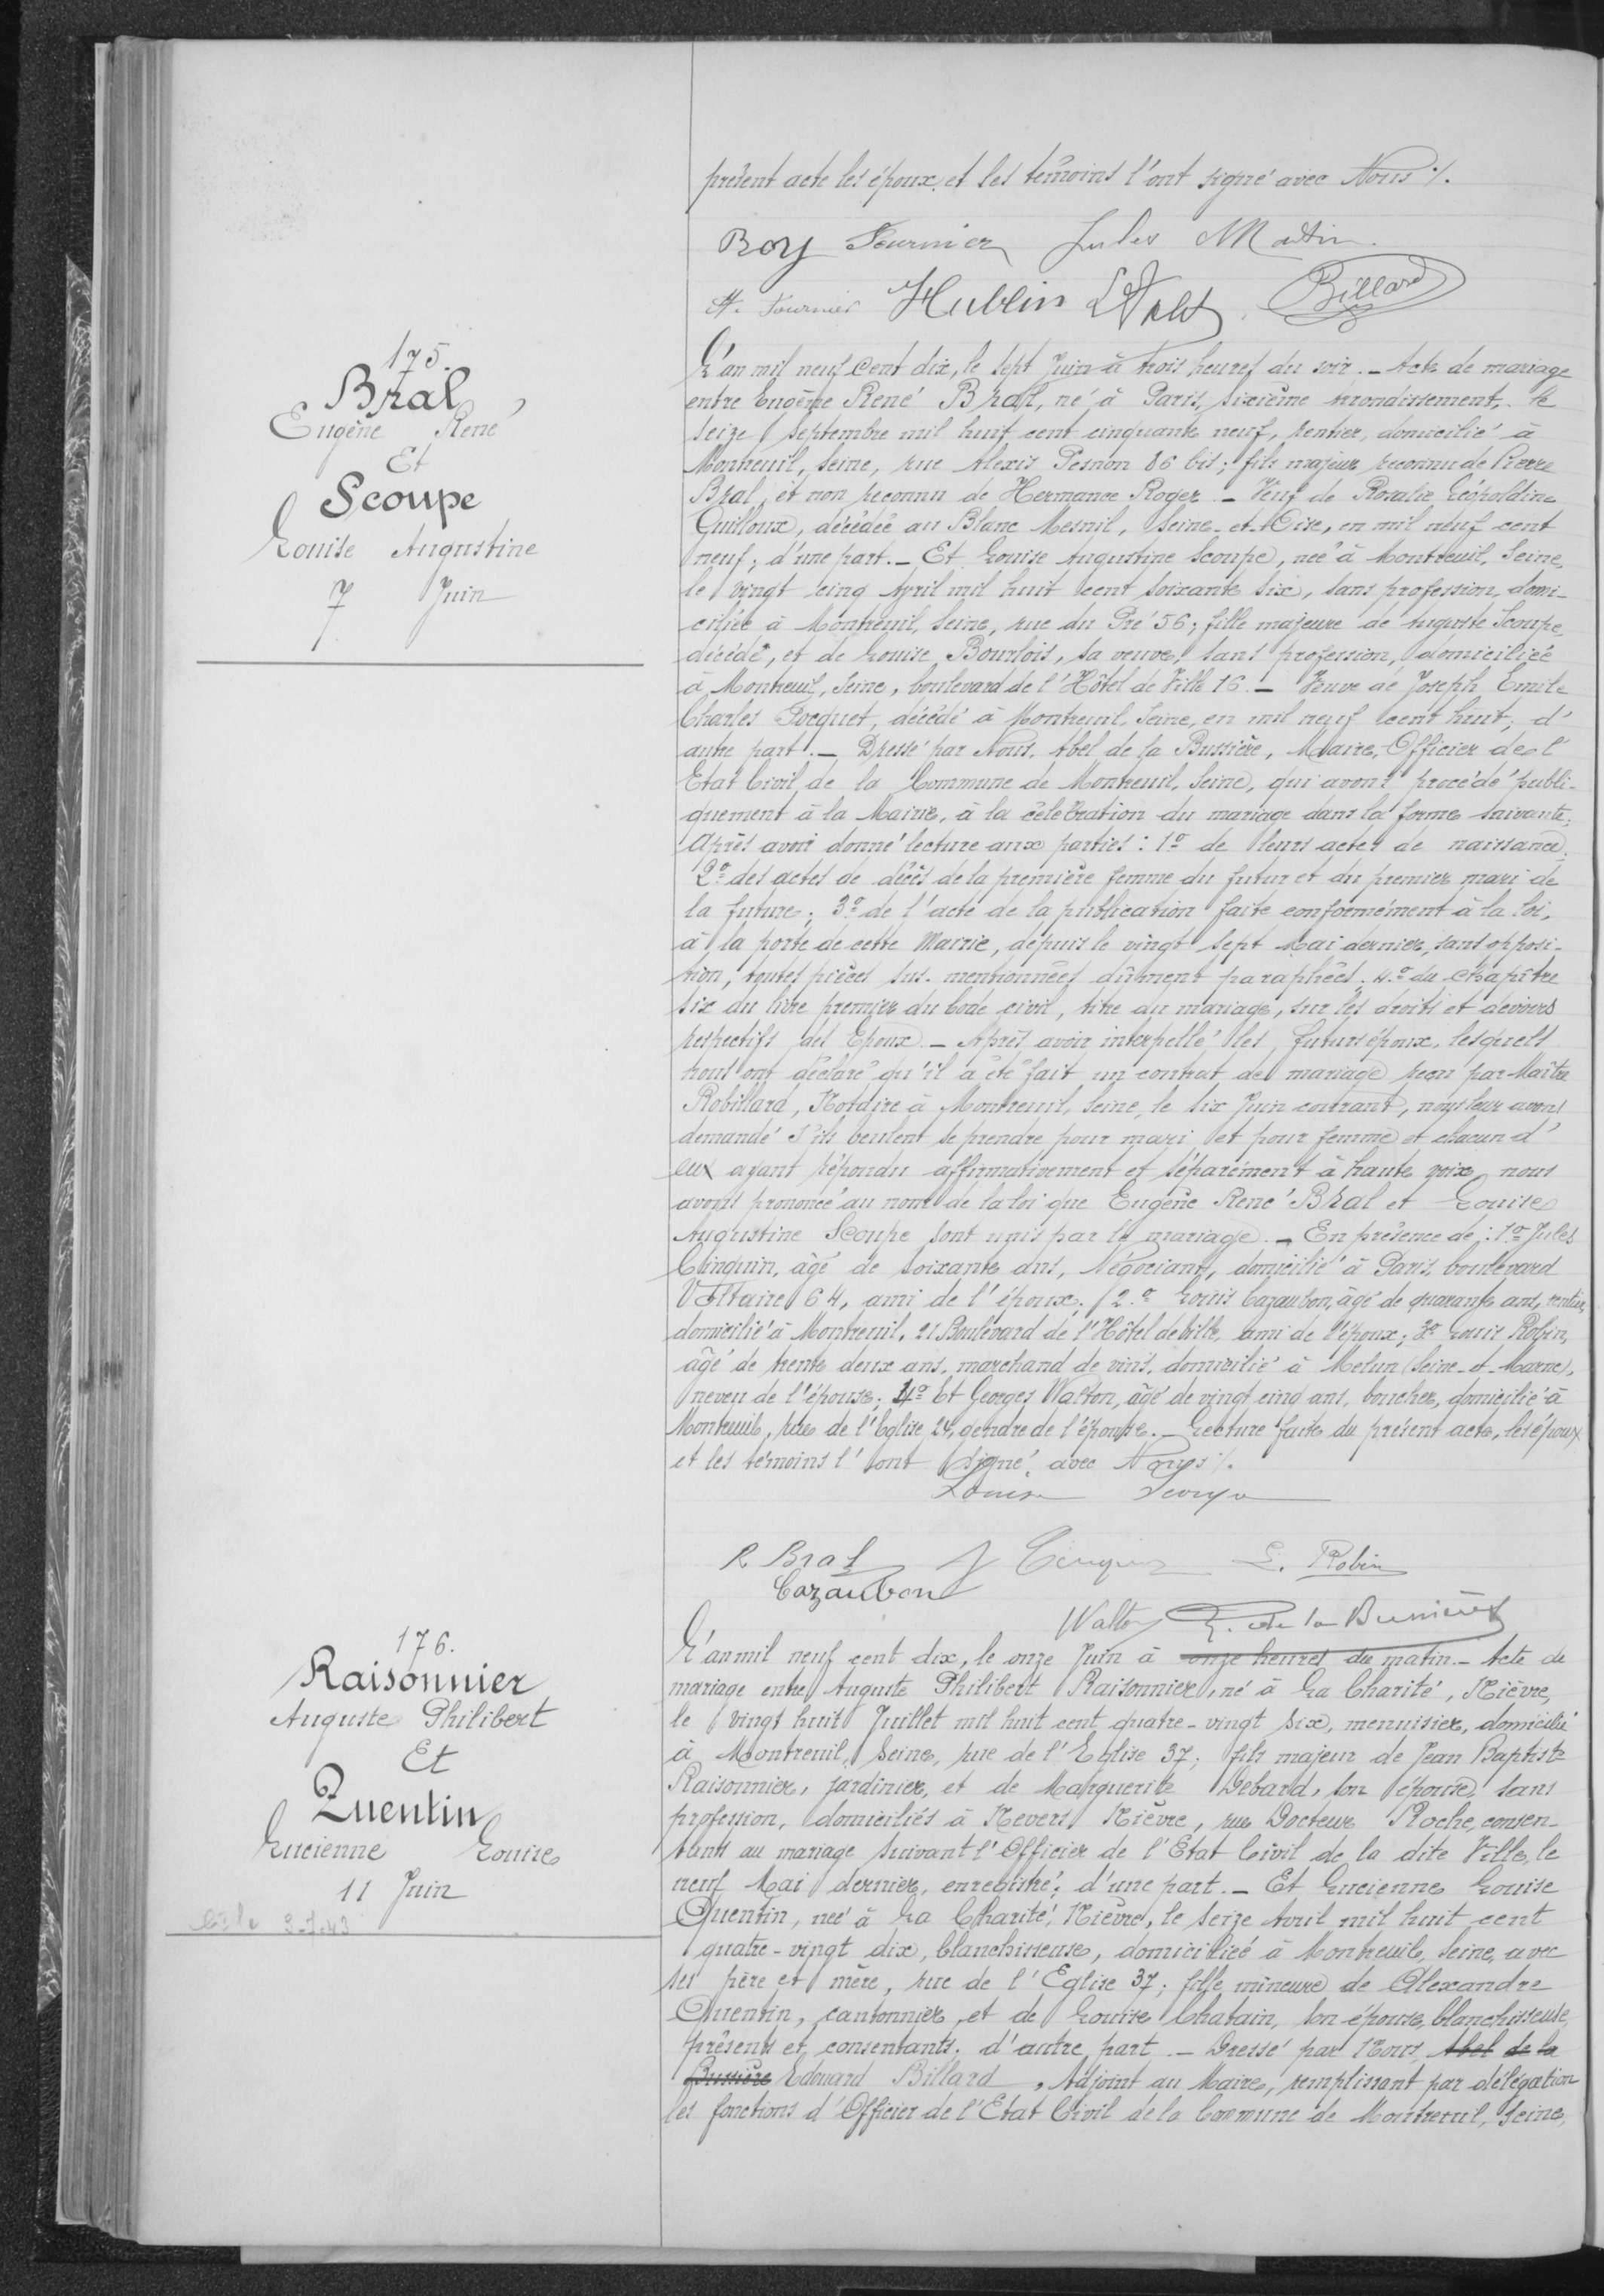175.
Bral
Eugène René
Et
Scoupe
Louise Augustine
7 Juin
L'an mil neuf cent dix, le sept Juin à trois heures du soir-Acte de mariage
entre Eugène René Bral, né à Paris, sixième arrondissement, le
seize septembre mil huit cent cinquante neuf, tennier, domicilié à
Montreuil, seine, rue Alexis Peson 86 bis; fils majeur reconnu de Pierre
Bral, et non reconnu de Hermance Roger-Veuf de Rosalie Léopoldine
Emilloux, décédée au Blanc Mesnil, Seine et oise, en mil neuf cent
neuf; d'une part.-Et Louise Augustine Scoupe, née à Montreuil, seine,
le vingt cinq Avril mil huit cent soixante six, sans profession, domi-
ciliée à Montreuil, Seine, rue du Pré 56; fille majeure de Auguste Scoupe,
décédé, et de Louise Bourlois, sa veuve, sans profession, domiciliée
à Montreuil, Seine, boulevard de l'Hîtel de Ville 16-Veuve de Joseph Emile
Charles Focquet, décédé à Montreuil, Seine, en mil neuf cent huit, d'
autre part .-Dressé par Nous, Abel de la Bussière, Maire, Officier de l'
Etat Civil de la Commune de Montreuil, Seine, qui avons procédé publi-
quement à la Mairie, à la célébration du mariage dans la forme suivante;
Après avoir donné lecture aux parties: 1°-de leurs actes de naissance,
2°-des actes de décès de la première femme du futur et des premier mari de
la future; 3°-de l'acte de la publication faite conformément à la loi,
à la porte de cette Mairie, depuis le vingt sept Mai dernier, sans opposi-
tion, toutes pièces sus-mentionnées dûment paraphées. 4°: du chapitre
six du livre premier du Code civil, titre du mariage, sur les droits et devoirs
respectifs des Epoux-Après avoir interpellé les futurs époux, lesquels
nous ont déclaré qu'il a été fait un contrat de mariage reçu par Maître
Robillard, notaire à Montreuil, Seine, le six Juin courant, nous leur avons
demandé s'ils veulent se prendre pour mari et pour femme et chacun d'
eux ayant répondu affirmativement et séparément à haute voix, nous
avons prononcé au nom de la loi que Eugène René Bral et Louise
Augustine Scoupe sont unis par le mariage-En présence de: 1°: Jules
Clinquin, âgé de soixante ans, Négociant, domicilié à Paris, boulevard
Voltaire 64, ami de l'époux; 2°/ Louis Cazaubon, âgé de quarante ans, rentier,
domicilié à Montreuil, 21 Boulevard de l'Hôtel de Ville, ami de l'époux; 3° Louis Robin,
âgé de trente deux ans, marchand de vins, domicilié à Melun (Seine-et-Marne),
neveu de l'époux; 4°: Et Georges Walton, âgé de vingt cinq ans, boucher, domicilié à
Montreuil, rue de l'Eglise 24, gendre l'épouse.-Lecture faite du présent acte, les époux
et les témoins l' ont signé avec Nous ./.
176
Raisonnier
Auguste Philibert
Et
Quentin
Lucienne Louise
11 Juin
L'an mil neuf cent dix, le onze Juin à onze heures du matin.-Acte de
mariage entre Auguste Philibert Raisonnier, né à la Charité, Nièvre,
le vingt huit Juillet, mil huit cent quatre-vingt dix, menuisier, domicilié
à Montreuil, Seine, rue de l'Eglise 37; fils majeur de Jean Baptiste
Raisonnier, jardinier, et de Marguerite Debard, son épouse, sans
profession, domiciliés à Nvers Nièvre, rue Docteur Roche, consen-
tants au mariage suivant l'Officier de l'Etat Civil de la dite Ville, le
neuf Mai dernier, enregistré d'une part .-Et Lucienne Louise
Quentin, née à la Charité, Nièvre, le seize Avril mil huit cent
quatre-vingt dix, blanchisseuse, domiciliée à Montreuil, Seine, avec
ses père et mère, rue de l'Eglise 37; fille mineure de Alexandre
Quentin, cantonnier, et de Louise Chatain, son épouse, blanchisseuse,
présents et consentants, d'autre part .-Dressé par Nous,
Edouard Billard, Adjoint au Maire, remplissant par délégation
les fonctions d'Officier de l'Etat Civil de la Commune de Montreuil, Seine,
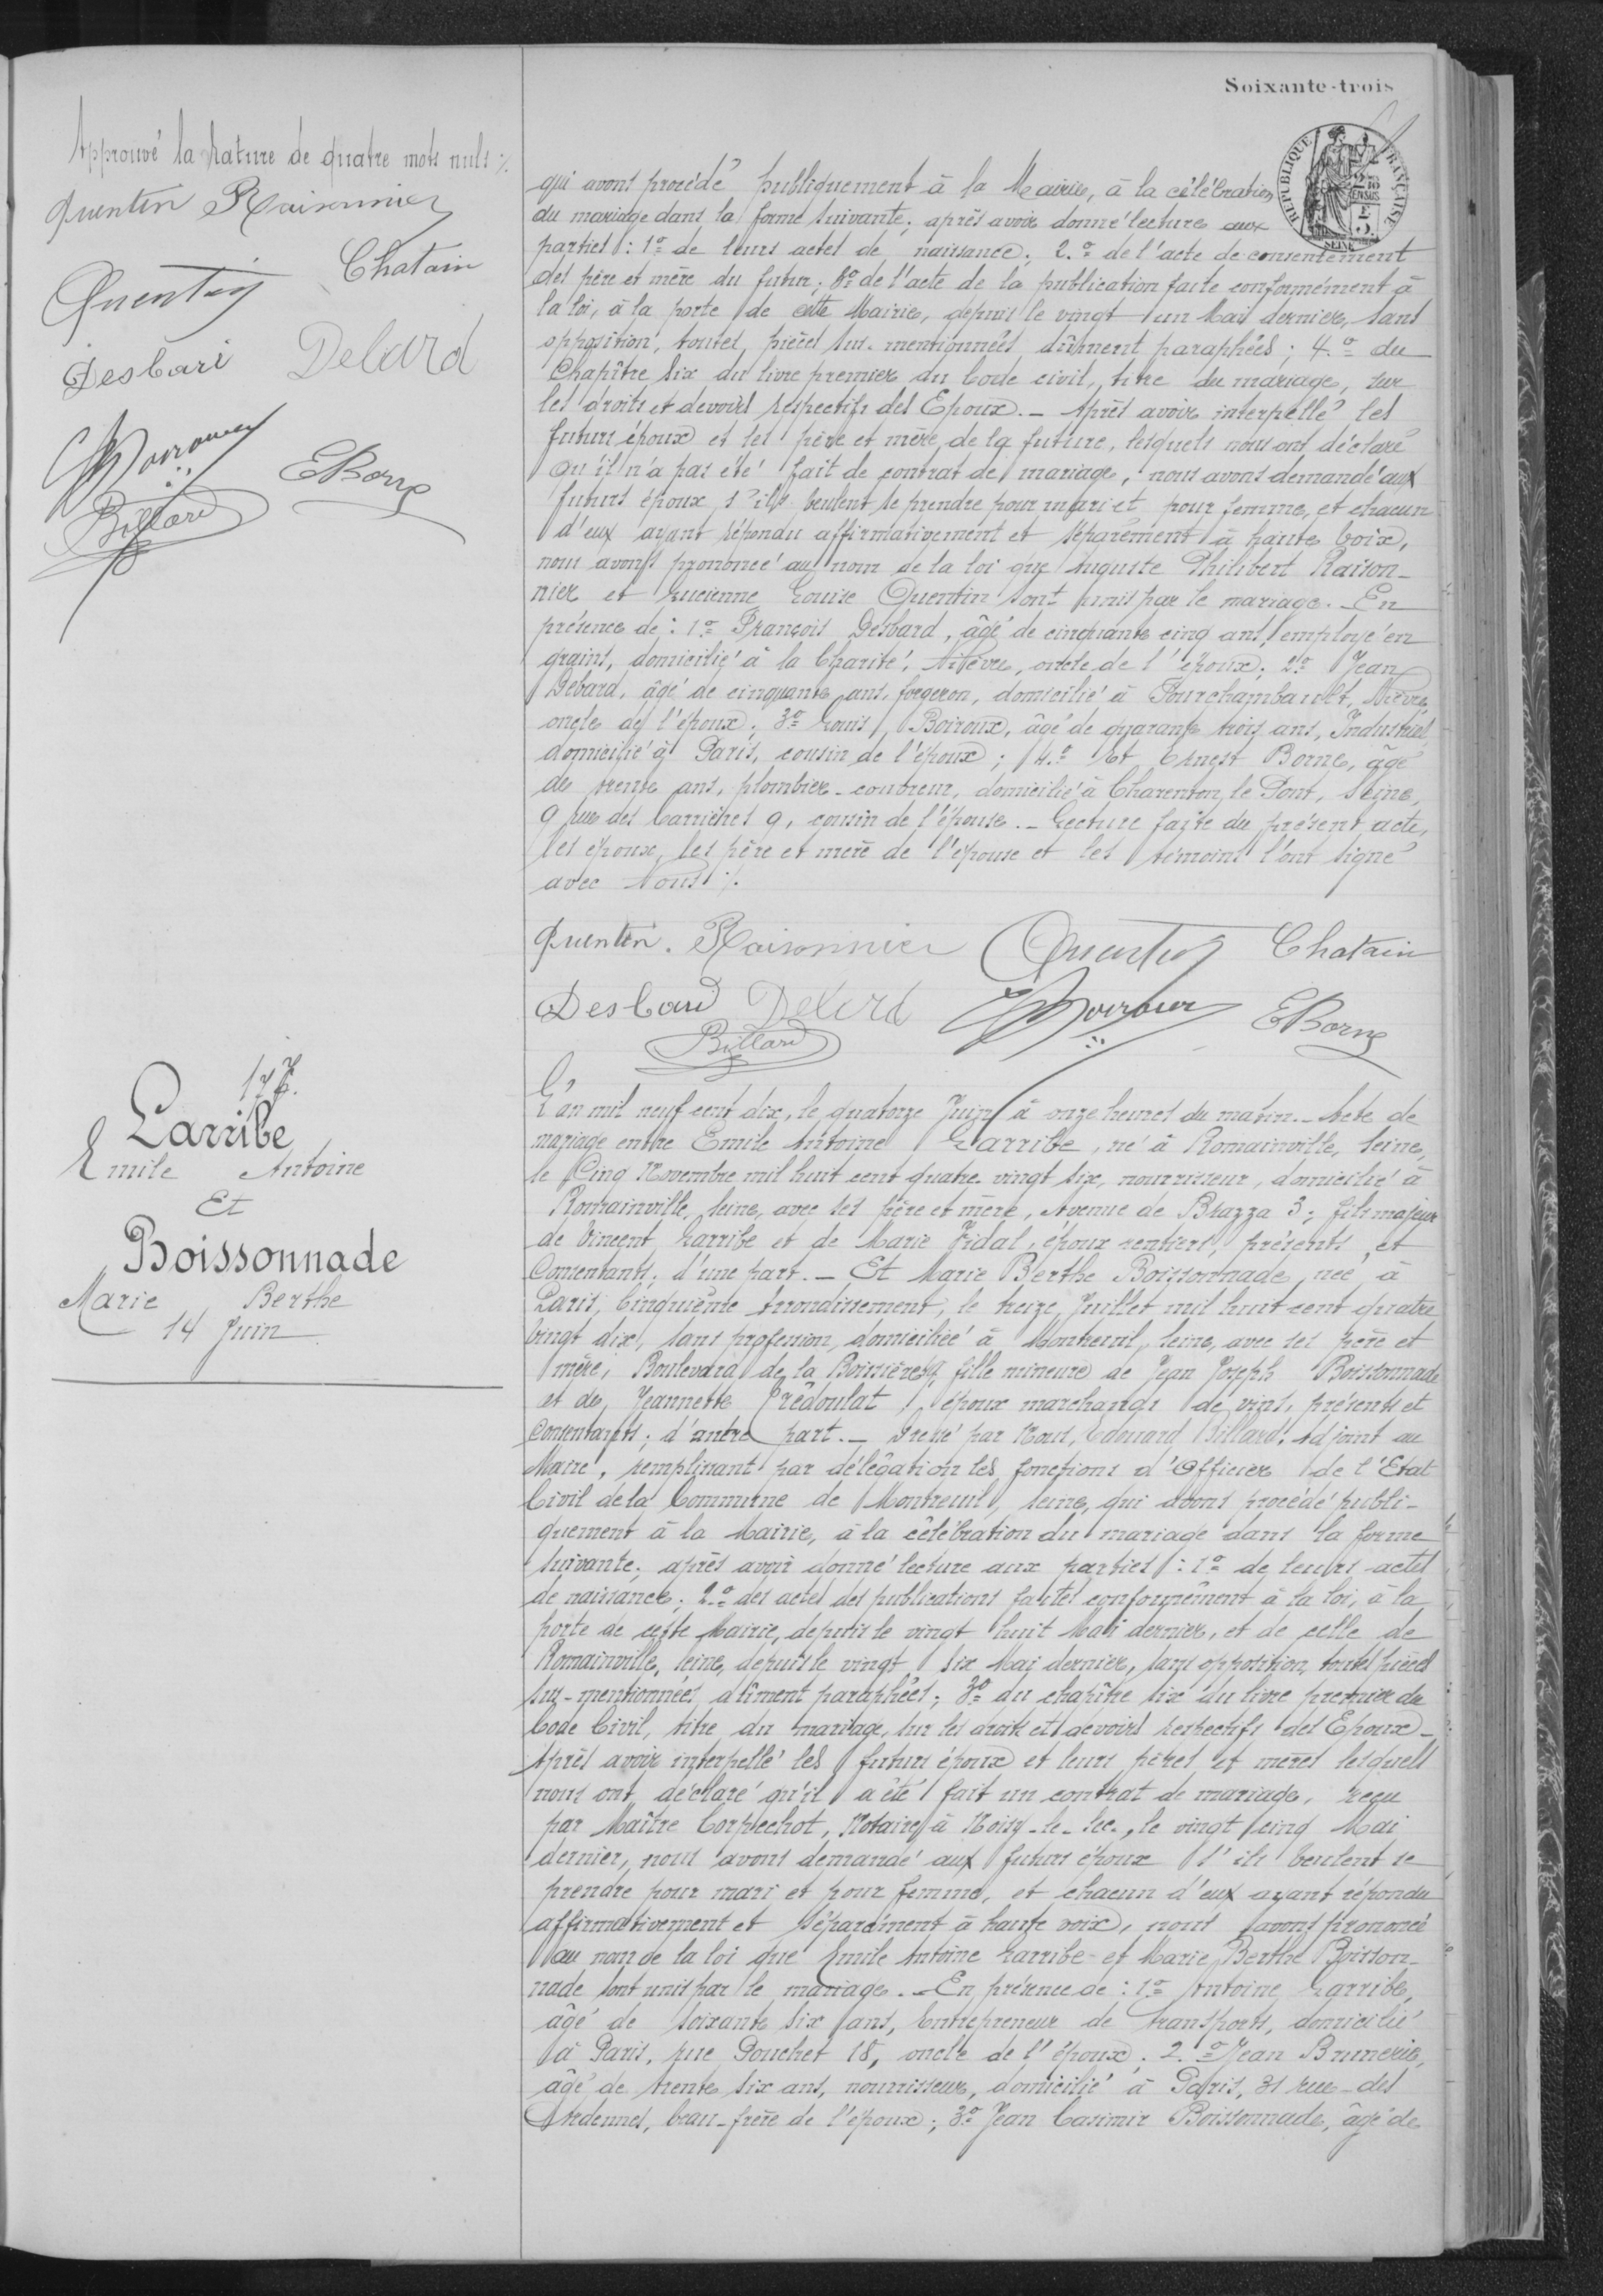qui avons procédé publiquement à la Mairie, à la célébration
du mariage dans la forme suivante; après avoir donné lecture aux
parties: 1°: de leurs actes de naissance, 2°: de l'acte de consentement
des père et mère du futur, 3°: de l'acte de la publication faite conformément à
la loi, à la porte de cette Mairie, depuis le vingt un Mai dernier, sans
opposition, toutes pièces sus-mentionnées dûment paraphées; 4°: du
Chapitre six du livre premier du Code civil, titre du mariage, sur
les droits et devoirs respectifs des Epoux.-Après avoir interpéllé les
futurs époux et les père et mère de la future, lesquels nous ont déclaré
qu'il n'a pas été fait de contrat de mariage, nous avons demandé aux
futurs époux s'ils veulent se prendre pour mari et pour femme, et chacun
d'eux ayant répondu affirmativement et séparément à haute voix,
nous avons prononcé au nom de la loi que Auguste Philibert Raison-
nier et Lucienne Louise Quentin sont unis par le mariage. En
présence de: 1°: François Desbard, âgé de cinquante cinq ans employé en
grains, domicilié à la Charité, Nièvre, oncle de l'époux; 2°: Jean
Debard, âgé de cinquante ans, forgeron, domicilié à Fourchambault, Nièvre,
oncle de l'époux; 3°: Louis Boiroux, âgé de quarante trois ans, Industriel
domicilié à Paris, cousin de l'époux; 4° et Ernest Bonne, âgé
de trente ans, plombier couvreur, domicilié à Charenton le Pont, Seine,
9 rue des Carrières 9, cousin de l'épouse.-lecture faite du présent acte;
les époux, les père et mère de l'épouse et les témoins l'ont signé
avec Nous ./.
177
Larribe
Emile Antoine
Et
Boissonnade
Marie Berthe
14 Juin
L'an mil neuf cent dix, le quatorze Juin à onze heures du matin. Acte de
mariage entre Emile Antoine Larribe, né à Romainville, Seine,
le Cinq Novembre mil huit cent quatre vingt six, nourrisseur, domicilié à
Romainville, Seine, avec ses père et mère, Avenue de Brazza 3. fils majeur
de Vincent Larribe et de Marie Fridal, époux rentiers, présents et
consentants; d'une part.-Et Marie Berthe Boissonnade née à
Paris, cinquième arrondissement; le treize Juillet mil huit cent quatre
vingt dix, sans profession, domiciliée à Montreuil, Seine, avec ses père et
mère; Boulevard de la Boissière, fille mineure de Jean Joseph Boissonnade
et de Jeannette Prédoulat, l'époux marchand de vins, présents et
consentants; d'autre part.-Dressé par Nous, Edouard Billard, Adjoint au
Maire, remplissant par délégation les fonctions d'Officier de l'Etat
Civil de la Commune de Montreuil, seine, qui avons procédé publi-
quement à la Mairie, à la célébration du mariage dans la forme
suivante. après avoir donné lecture aux parties: 1°: de leurs actes
de naissance; 2°: des actes des publications faites conformément à la loi, à la
porte de cette Mairie, depuis le vingt six Mai dernier, et de celle de
Romainville, Seine, depuis le vingt six Mai dernier, sans opposition, toutes pièces
sus-mentionnées dûment paraphées; 3°: du chapitre six du livre premier du
Code Civil, titre du mariage, sur les droits et devoirs respectifs des Epoux.
Après avoir interpellé les futurs époux et leurs pères et mères lesquels
nous ont déclaré qu'il a été fait un contrat de mariage, reçu
par Maître Corpechot, Notaire à Noisy-le-Sec, le vingt cinq Mai
dernier, nous avons demandé aux futurs époux s'ils veulent se
prendre pour mai et pour femme, et chacun d'eux ayant répondu
affirmativement et séparément à haute voix, nous avons prononcé
au nom de la loi que Emile Antoine Larribe et Marie Berthe Boisson
nade sont unis par le mariage. En présence de: 1° Antoine Larribe,
âgé de soixante six ans, entrepreneur de transports, domicilié
à Paris, rue Pouchet 18, oncle de l'époux; 2°: Jean Brunerie
âgé de trente six ans, nourrisseur, domicilié à Paris, 31 rue des
Ardennes, beau-frère de l'époux; 3°: Jean Casimir Boissonnade, âgé de